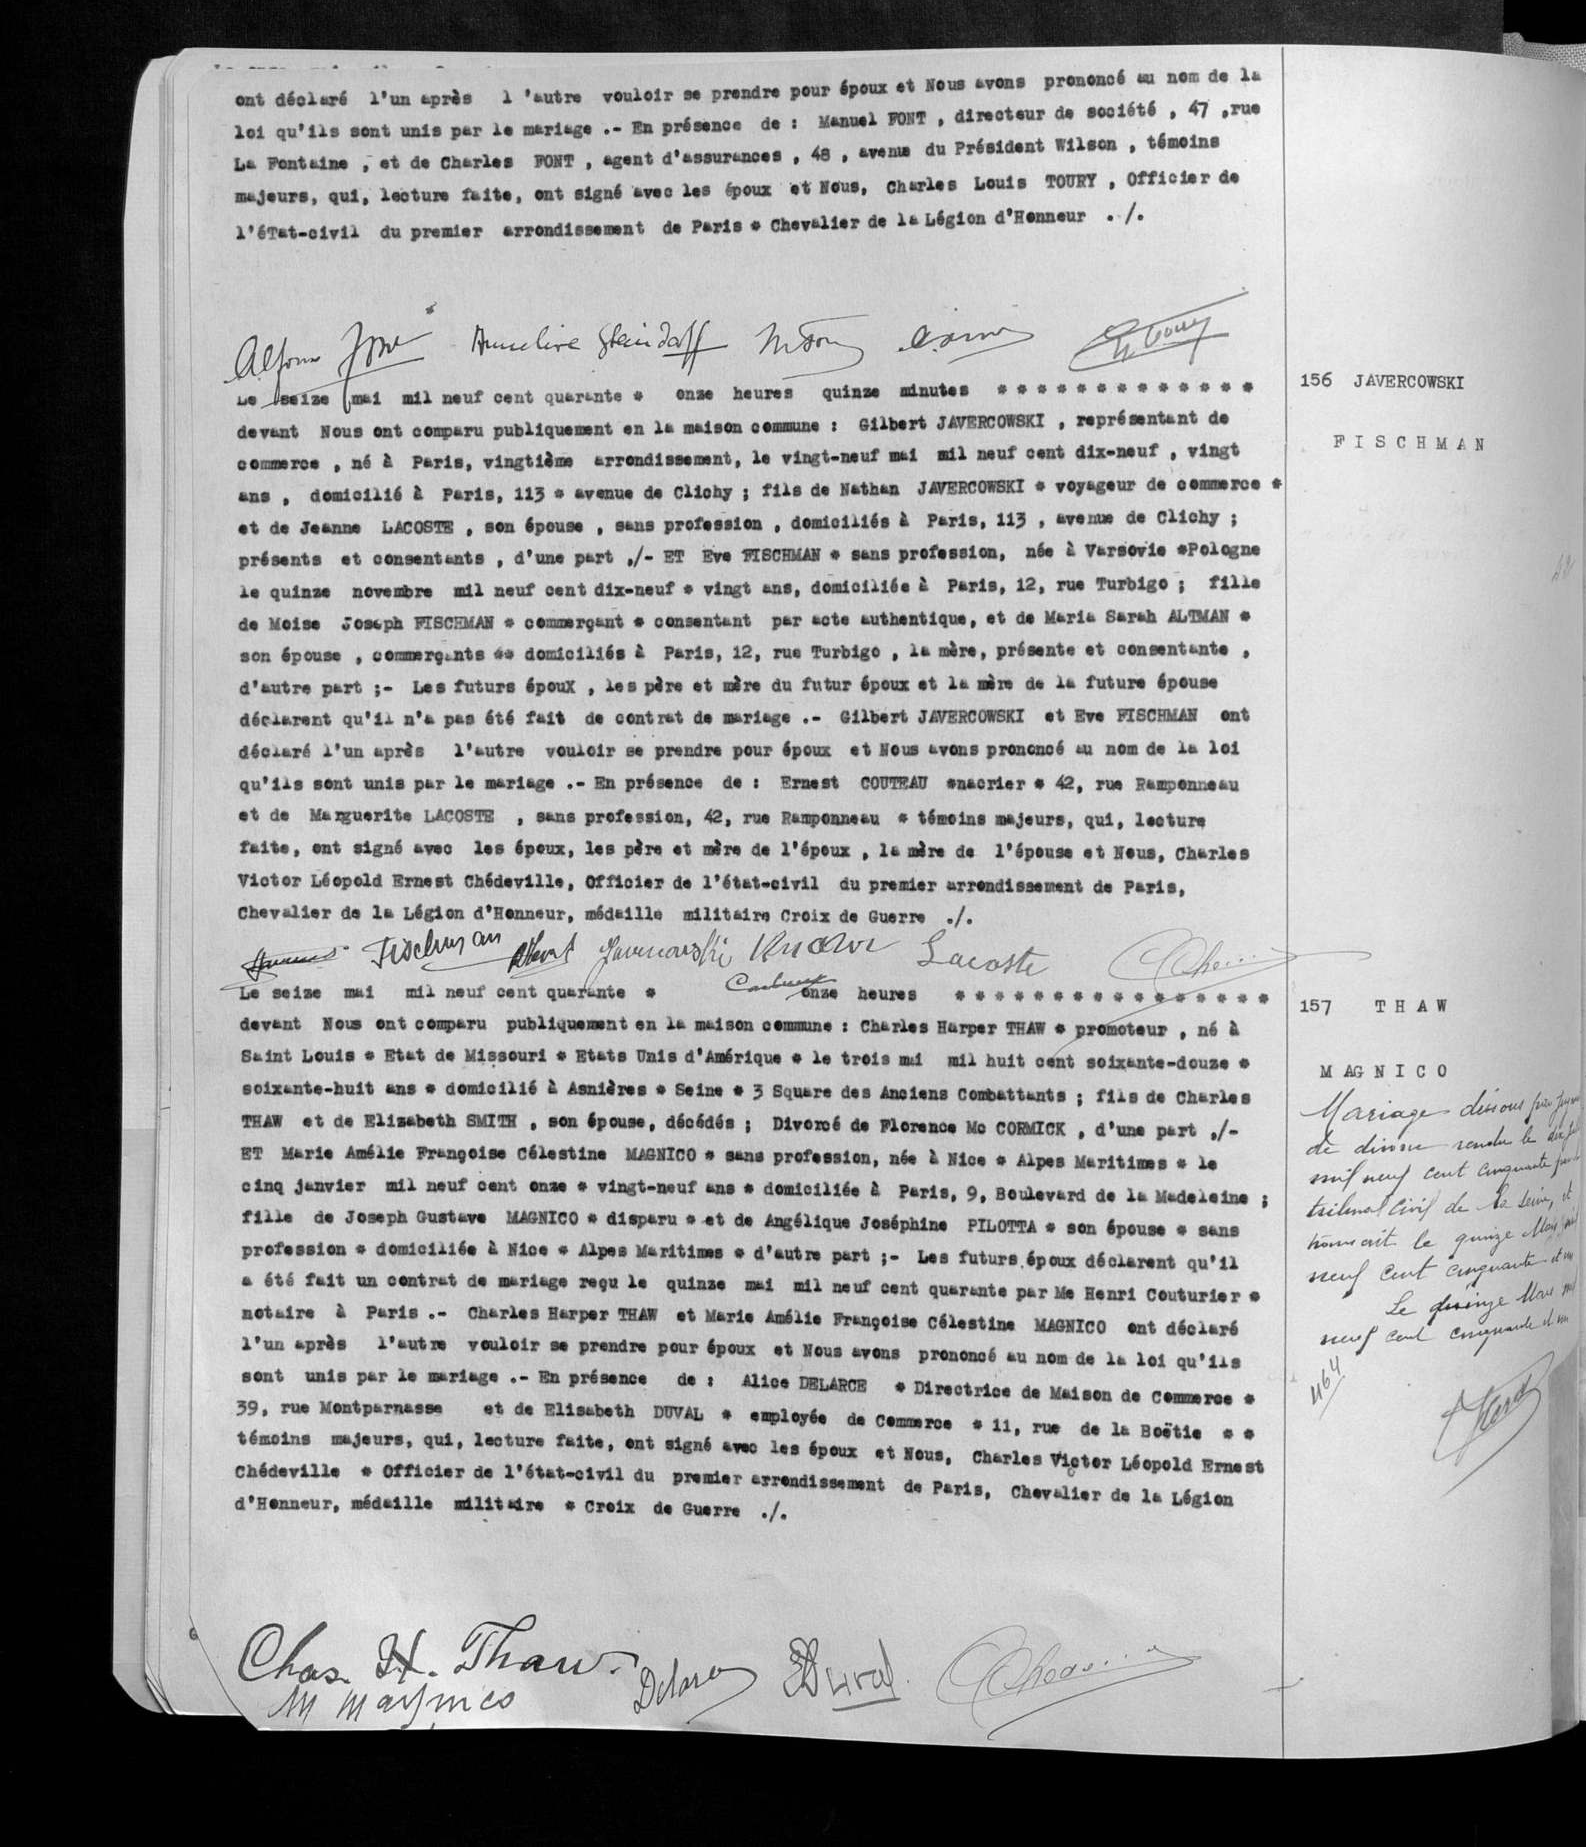Ont déclaré l'un après l'autre vouloir se prendre pour époux et Nous avons prononcé au nom de la
loi qu'ils sont unis par le mariage .-En présence de; Manuel FONT, directeur de société, 47, rue
La Fontaine, et de Charles FONT, agent d'assurances, 48, avenue du Président Wilson, témoins
majeurs, qui, lecture faite, ont signé avec les époux et Nous, Charles Louis TOURY, Officier de
l'éTat-civil du premier arrondissement de Paris * Chevalier de la Légion d'Honneur ./.
Le seize mai mil neuf cent quarante * onze heures quinze minutes ****
devant Nous ont comparu publiquement en la maison commune: Gilbert JAVERCOWSKI, représentant de
commerce, né à Paris, vingtième arrondissement, le vingt-neuf mai mil neuf cent dix-neuf, vingt
ans, domicilié à Paris, 113 * avenue de Clichy; fils de Nathan JAVERCOWSKI * voyageur de commerce *
et de Jeanne LACOSTE, son épouse, sans profession, domiciliés à Paris, 113, avenue de Clichy;
présents et consentants, d'une part ,/-ET Eve FISCHMAN * sans profession, née à Varsovie * Pologne
le quinze novembre mil neuf cent dix-neuf * vingt ans, domiciliée à Paris, 1é, rue Turbigo; fille
de Moise Joseph FISCHMAN * commerçant * consentant par acte authentique, et de Maria Sarah ALTMAN *
son épouse, commerçants ** domiciliés à Paris, 12, rue Turbigo, la mère, présente et consentante,
d'autre part ;-Les futurs époux, les père et mère du futur époux et la mère de la future épouse
déclarent qu'il n'a pas été fait de contrat de mariage .-Gilbert JAVERCOWSKI et de Eve FISCHMAN ont
déclaré l'un après l'autre vouloir se prendre pour époux et Nous avons prononcé au nom de la loi
qu'ils sont unis par le mariage .-En présence de: Ernest COUTEAU * nacrier * 42, rue Ramponneau
et de Marguerite LACOSTE, sans profession, 42, rue Ramponneau * témoins majeurs, qui, lecture
faite, ont signé avec les époux, les père et mère de l'époux, la mère de l'épouse et Nous, Charles
Victor Léopold Ernest Chédeville, Officier de l'état-civil du premier arrondissement de Paris,
Chevalier de la Légion d'Honneur, médaille militaire Croix de Guerre ./.
Le seize mai mil neuf cent quarante * onze heures ****
devant Nous ont comparu publiquement en la maison commune: Charles Harper THAW * promoteur, né à
Saint Louis * Etat de Missouri * Etats Unis d'Amérique * le trois mai mil huit cent soixante-douze *
soixante-huit ans * domicilié à Asnières * Seine * 3 Square des Anciens Combattants; fils de Charles
THAW et de Elisabeth SMITH, son épouse, décédés; Divorcé de Florence Mo CORMICK, d'une part ,/
ET Marie Amélie Françoise Célestine MAGNICO * sans profession, née à Nice * Alpes Maritimes * le
cinq janvier mil neuf cent onze * vingt-neuf ans * domiciliée à Paris, 9, Boulevard de la Madeleine;
fille de Joseph Gustave MAGNICO * disparu * et de Angélique Joséphine PILOTTA * son épouse * sans
profession * domiciliée à Nice * Alpes Maritimes * d'autre part ;-Les futurs époux déclarent q'iil
a été fait un contrat de mariage reçu le quinze mai mil neuf cent quarante par Me Henri Couturier *
notaire à Paris .-Charles Harper THAW et Marie Amélie Françoise Célestine MAGNICO ont déclaré
l'un après l'autre vouloir se prendre pour époux et Nous avons prononcé au nom de la loi qu'ils
sont unis par le mariage .-En présence de: Alice DELARCE * Directrice de la Maison de Commerce *
39, rue Montparnasse et de Elisabeth DUVAL * employée de Commerce * 11, rue de la Boëtie **
témoins majeurs, qui, lecture faite, ont signé avec les époux et Nous, Charles Victor Léopold Ernest
Chédeville * Officier de l'état-civil du premier arrondissement de Paris, Chevalier de la Légion
d'Honneur, médaille militaire * Croix de Guerre ./.
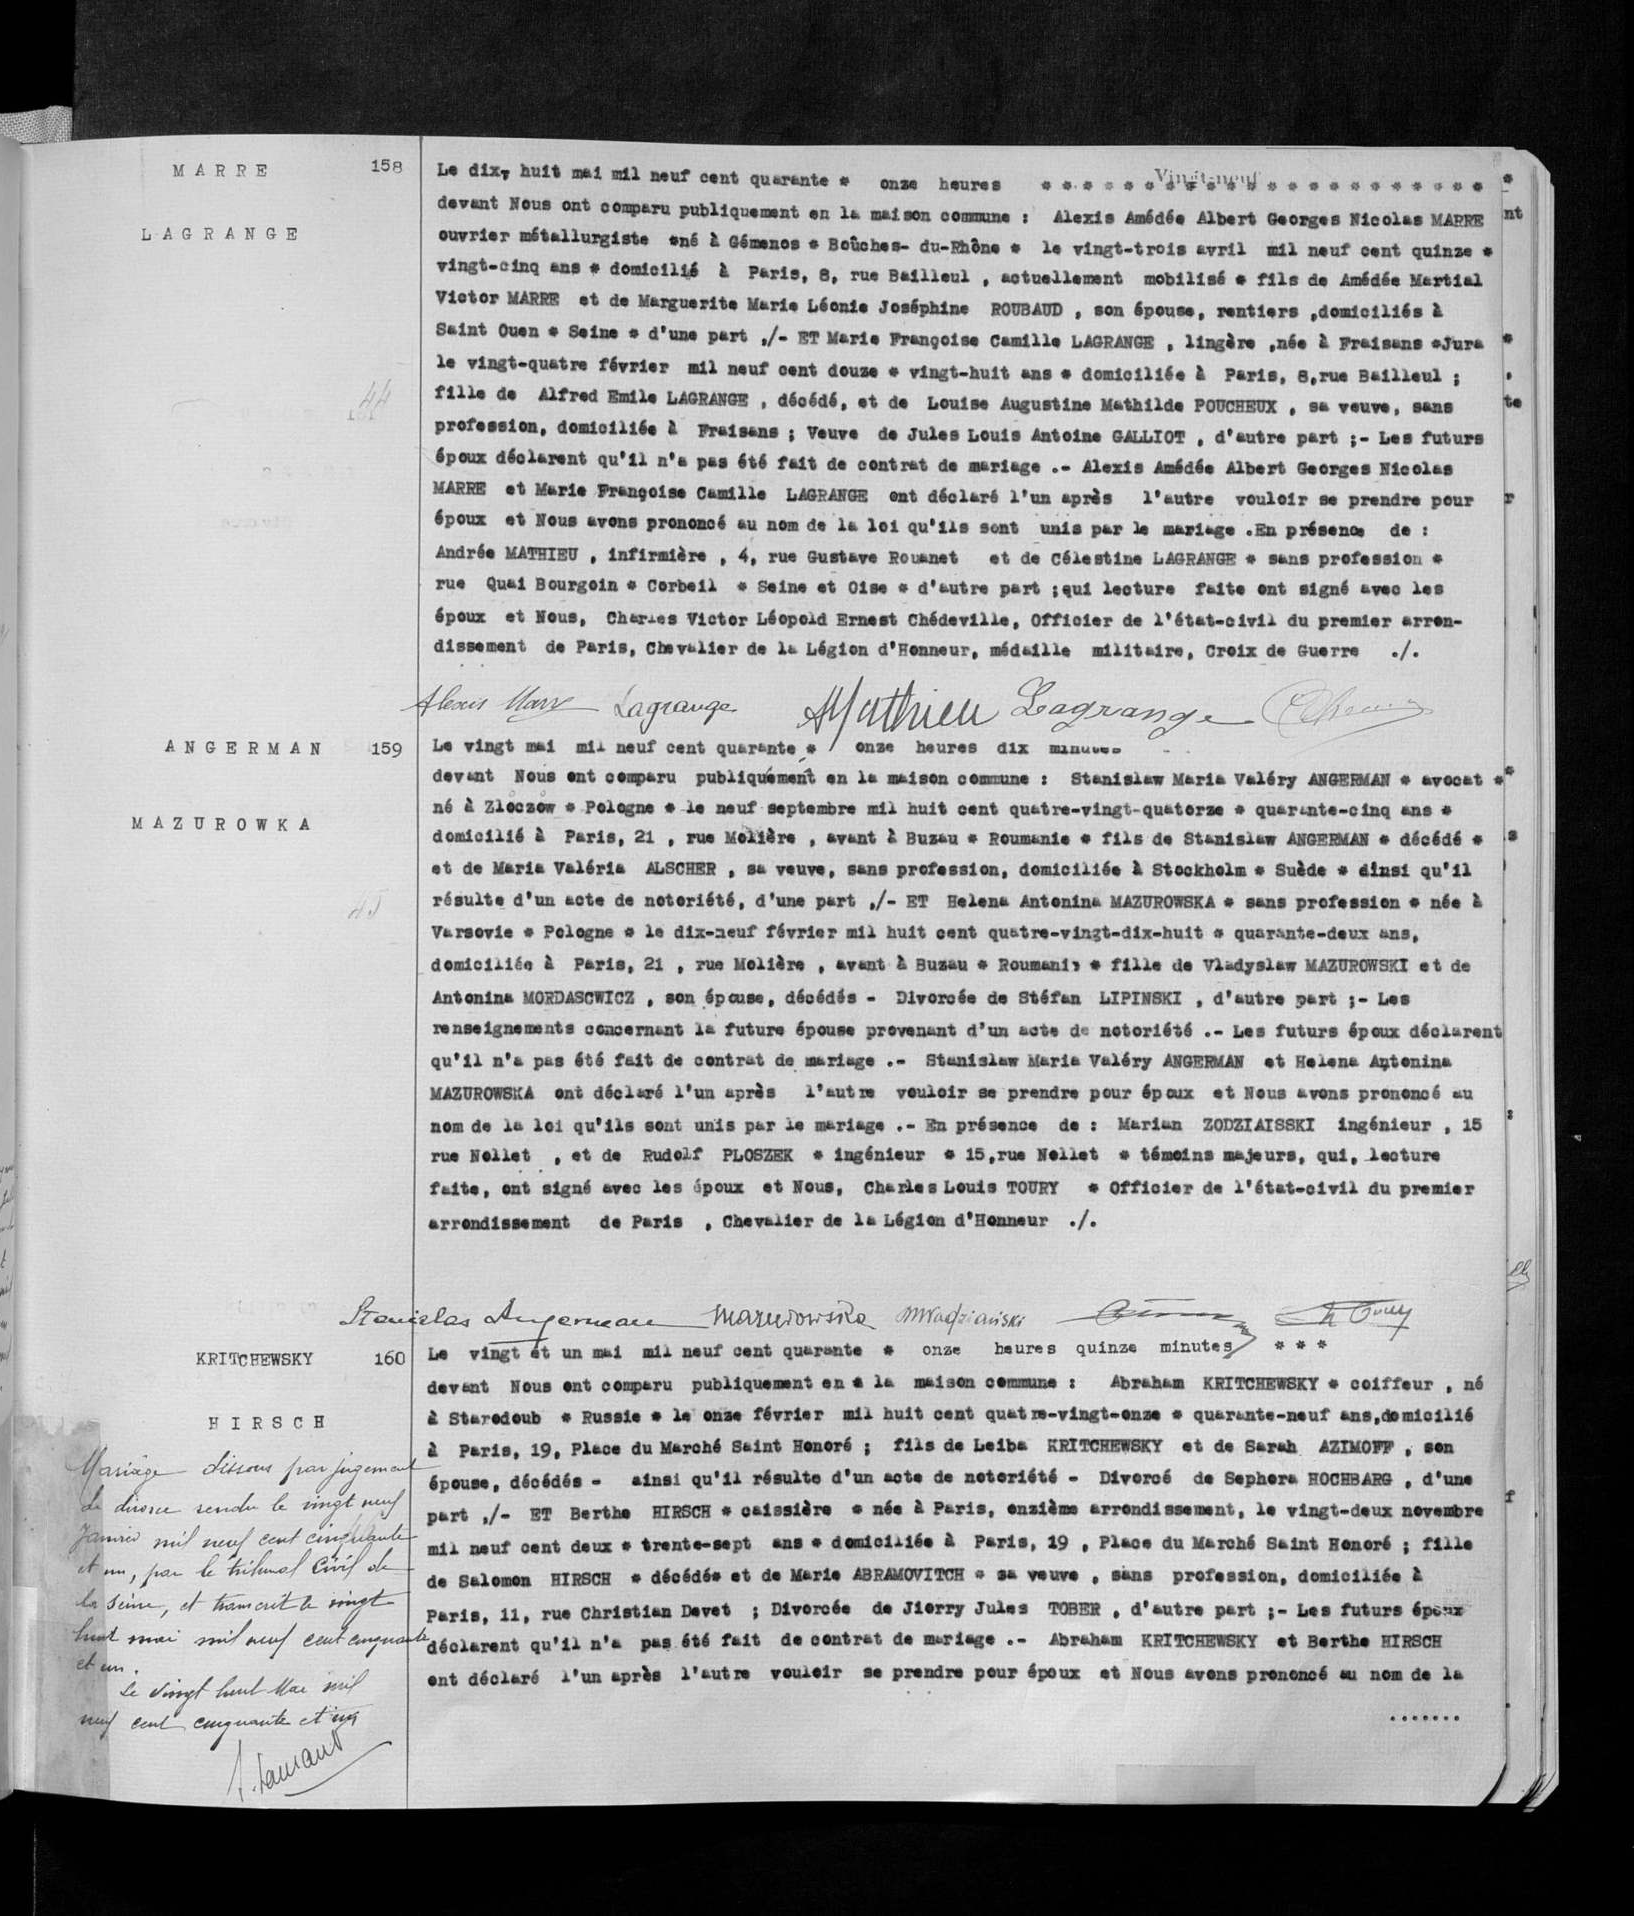Le dix huit mai mil neuf cent quarante * onze heures ****
devant Nous ont comparu publiquement en la maison commune: Alexis Amédée Albert Georges Nicolas MARRE
ouvrier métallurgiste * né à Gémenos * Boûches-du-Rhône * le vingt-trois avril mil neuf cent quinze *
vingt-cinq ans * domicilié à Paris, 8, rue Bailleul, actuellement mobilisé * fils de Amédée Martial
Victor MARRE et de Marguerite Marie Léonie Joséphine ROUBAUD, son épouse, rentier, domiciliés à
Saint Ouen * Seine * d'une part ,/-ET Marie Françoise Camille LAGRANGE, lingère, née à Fransans * Jura
le vingt-quatre février mil neuf cent douze * vingt-huit ans * domicilié à Paris, 8, rue Bailleul;
fille de Alfred Emile LAGRANGE, décédé, et de Louise Augustine Mathilde POUCHEUX, sa veuve, sans
profession, domiciliée à Fraisans; Veuve de Jules Louis Antoine GALLIOT, d'autre part ;-Les futurs
époux déclarent qu'il n'a pas été fait de contrat de mariage .-Alexis Amédée Albert Georges Nicolas
MARRE et Marie Françoise Camille LAGRANGE ont déclaré l'un après l'autre vouloir se prendre pour
époux et Nous prononcé au nom de la loi qu'ils sont unis par le mariage. En présencee de;
Andrée MATHIEU, infirmière, 4, rue Gustave RUANET et de Célestine LAGRANGE * sans profession *
rue Quai Bourgoin * Corbeil * Seine et Oise * d'autre part; qui lecture faite ont signé avec les
époux et Nous, Charles Victor Léopold Ernest Chédeville, Officier de l'état-civil du premier arron-
dissement de Paris, Chevalier de la Légion d'Honneur, médaille militaire, Croix de Guerre ./.
Le vingt mai mil neuf cent quarante * onze heures dix minutes
devant Nous ont comparu publiquement en la maison commune: Stanislaw Maria Valéry ANGERMAN * avocat *
né à Zloczow * Pologne * le neuf septembre mil huit cent quatre-vingt-quatorze * quarante-cinq ans *
domicilié à Paris, 21, rue Molière, avant à Buzau * Roumanie * fils de Sanislaw ANGERMAN * décédé *
et de Maria Valéria ALSCHEER, sa veuve, sans profession, domiciliée à Stockholm * Suède * ainsi qu'il
résulte d'un acte de notoriété, d'une part ,/-ET Helena Antonina MAZUROWSKA * sans profession * née à
Varsovie * Pologne * le dix-neuf février mil huit cent quatre-vingt-dix-huit * quarante-deux ans,
domiciliée à Paris, 21, rue Molière, avant à Buzau * Roumani * fille de Vladyslaw MAZUROWSKI et de
Antonina MORDASCWICZ, son épouse, décédés-Divorcée de Stéfan LIPINSKI, d'autre part ;-Les
renseignements concernant la future épouse provenant d'un acte de notoriété .-Les futurs époux déclarent
qu'il n'a pas été fait de contrat de mariage .-Stanislaw Maria Valéry ANGERMAN et Helena Antonina
MAZUROWSKA ont déclaré l'un après l'autre vouloir se prendre pour époux et Nous avons prononcé au
nom de la loi qu'ils sont unis par le mariage .-En présence de: Marian ZODZIAISSKI ingénieur, 15
rue Nollet, et de Rudolf PLOSZEK * ingénieur * 15, rue Nollet * témoins majeurs, qui, lecture
faite, ont signé avec les époux et Nous, Charles Louis TOURY * Officier de l'état-civil du premier
arrondissement de Paris, Chevalier de la Légion d'Honneur ./.
Le vingt et un mai mil neuf cent quarante * onze heures quinze minutes ****
devant Nous ont comparu publiquement en * la maison commune: Abraham KRITCHEWSKY * coiffeur, né
à Staredoub * Russie * le onze février mil huit cent quatre-vingt-onze * quarante-neuf ans, domicilié
à Paris, 19, Place du Marché Saint Honoré; fils de Leiba KRITCHEWSKY et de Sarah AZIMOFF, son
épouse, décédés-ainsi qu'il résulte d'un acte de notoriété-Divorcé de Sephora HOCHBARG, d'une
part ,/-ET Berthe HRISCH * caissière * née à Paris, onzième arrondissement, le vingt-deux novembre
mil neuf cent deux * trente-sept ans * domiciliée à Paris, 19, Place du Marché Saint Honoré; fille
de Salomon HIRSCH * décédé * et de Marie ABRAMOVITCH * sa veuve, sans profession, domiciliée à
Paris, 11, rue Christian Devet; Divorcée de Jierry Jules TOBER, d'autre part ;-Les futurs époux
déclarent qu'il n'a pas été fait de contrat de mariage .-Abraham KRITCHEWSKY et Berthe HIRSCH
ont déclaré l'un après l'autre vouloir se prendre pour époux et Nous avons prononcé au nom de la